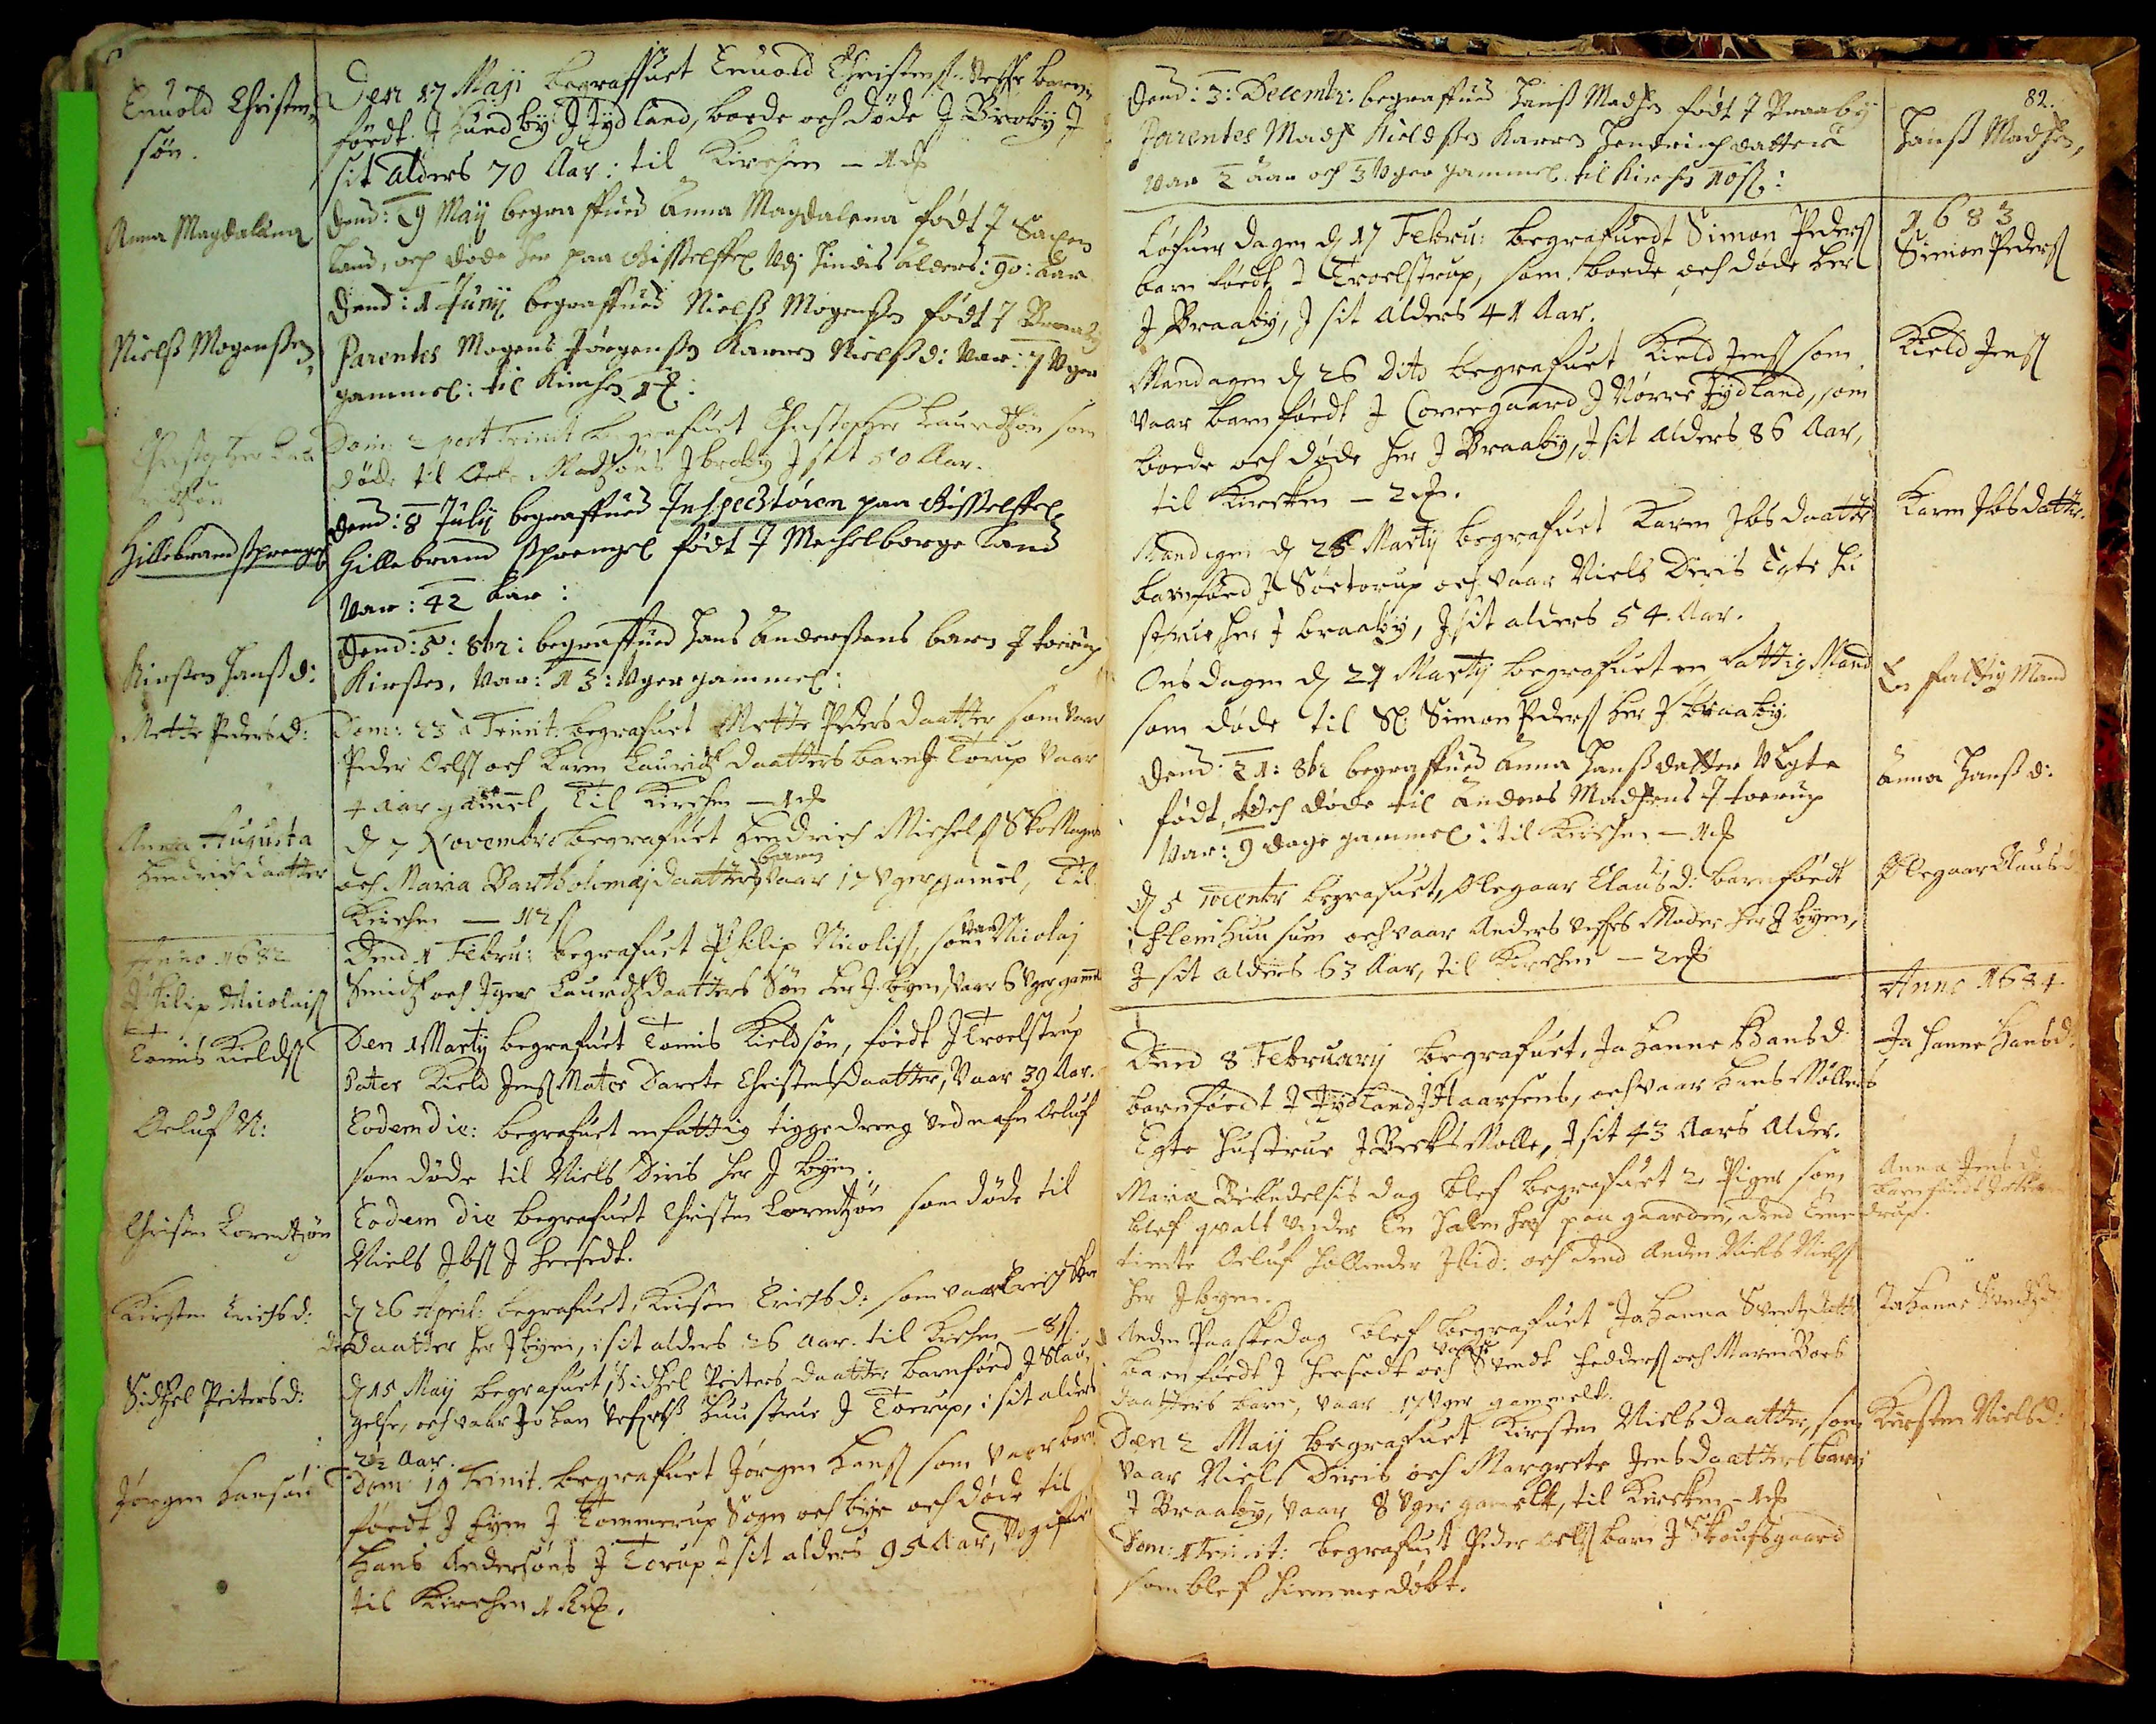Envold Christen¬
søn 
Den 17 May begraffuet Envold Christens: Veffer barn
føedt i Lundby J Jydland, boede och døde J Braaby. J
sit alders 70 Aar: til Kirchen 1 Mk.
Anna Magdalene
Dend: 29 May begraffued Anna Magdalene født J Saxens
Land, och døde her paa Gisselffel Udj hindis Alders: 90: Aar. 
Dend: 1 Juny begraffued Niels Mogensen født J Braaby
Niels Mogensen
Parentes Mogens Jørgensøn Karren NielsD: Var: 7 Uger 
gammel: til Kirchen 1 Mk
Christopher Laurid-
zøn
Dom 2 post Trinit begrafuet Christopher Lauridsøn som
døde til Oele Madzøns J Broby J sit 50 Aar.
Hillebrand Sprengel##
Dend: 8 July begraffued Inspecktøren paa Gisselffel
Hillebrand Sprengel født J Mechelborgs Land
var: 42 Aar:
Kirsten Hansd:
Dend: 5: 8br: begraffued Hans Andersøns Barn J Toerup;
Kirsten. Var: 13: Uger gammel:
Mette Pedersd:
Dom 23 á´Trinit: begrafuet Mette Pedersdaatter som vare
Peder Oels och Karen Lauridzdaatters Barn J Torup. Vaar
4 Aar gammel, til Kirchen 1Mk.
Anna## Augusta
Hendrichdaatter 
D. 7. November begrafuet Hendrich Michels SkoMager
Barn
och Maria Bartholemæj Daatters. Vaar 17 Uger gammel. Til
Kirchen – 12 Sk.
Anno 1682
Philip Nicolais
Den 1 Febru: begrafuet Philip Nicolis, som var Nicolaj
Smidz och Jnger Lauridzdaatters Søn her J Byen. Vaar 6 Uger Gammel.
Tomis Kields
Den 1 Marty begrafuet Tomis Kieldsøn, føedt J Troelstrup.
Pater Kield Jens, Mater Dorete Christensdaatter. Vaar 39 Aar.
Oeluf N:
Eodem die: begrafuet en fattig Tiggedreng ved Nafn Oeluf
som døde til Niels Diris her J Byen.
Christen Lorentzøn
Eodem die begrafuet Christen Lorentzøn som døde til
Niels Jbs J Hersedt.
Kirsten Erichsd:
D. 26 April: begraffuet Kirsten ErichsD: som vaar Erich ##
ders## Daatter her J byen, i sit alders 26 Aar, til Kirchen - 8 Sk:
Sidzel Pedersd. 
D. 15 May begrafuet, Sidzel Peitersdaatter barnføed J Slau¬
gelse, och vaar Johan Vesztes## Hustrue J Toerup, i J sit alders
20 ½ Aar.
Jørgen Hansøn
Dom: 19 Trinit. begrafuet Jørgen Hans som vaar barn
føedt J Fyen J Tommerup Sogn och bye och døde til
Hans Andersøns J Torup J sit alders 95 Aar, udgift
til Kirchen 1 Rmk.
Dend: 3: Decembr: begraffued Hans Madsen født J Braaby.
Parentes Mads Nielssen, Karen Hendrichdatter,
var 2 Aar och 3 Uger gammel, til Kirche 10 Sk:
82.
Hans Madsen
Løfensdagen d. 17 Febru: Begraffuedt Simon Peders
barn føedt J Troelstrup, som boede och døde her
J Braaby, J sit alders 41 Aar. 
1683
Simon Pedersøn
Mandagen d 26 dito begrafuet Kield Jens som
vaar barn føedt J Corregaard J Nørre Jydland, som
boede och døde her J Braaby, J sit alders 86 Aar,
til Kircken - 2 Mk.
Kield Jens
Mandagen d. 26 Marty begrafuet Karen Jbsdaatter
barnføed J Søetorup och Vaar Niels Diris Egte Hu
strue her J Braaby, J sit alders 54 Aar.
Karen Jbsdaatter.
Onsdagen d. 27 Marty begrafuet en fattig Mand
som døde til SL: Simon Peders her J Braaby.
En fattig Mand
Dend: 21: 8br. . Begrafued Anna Hansdatter Uegte
født. och døde til Anders Madsens J Toerup,
Var: 9 Dage gammel: til Kirchen - 1 Mk.
Anna Hansd:
D. 5 10cembr. begrafued Ølegaar Clausd: Barnføedt
J  FlemmHuusum##  och vaar Anders Veffrs Moeder her J byen,
J sit alders 63 Aar, til Kirchen - 2 Mk.
Ølegaar Clausd:
Den 8 February begrafuet, Johanne HansD
barnføedt J Jydland J Haarsens, och vaar Hans Møllers
Egte Hustrue J BecksMølle, J sit 43 Aars Alder.
Anno 1684
Johanne Hansd:
Maria Bebudelsis Dag blef begrafuet 2 Piger som
blef qvalt under En Halm Hees## her paa gaarden, dend Eene
tiente Oeluf hollænder Jbid: och dend anden Niels Niels
her J byen.
Anna Jensd:
barnføedt i Otted##
drup##
Anden Paaskedag blef begrafuet Johanna Sventzdatter
vaar
barnføedt J Hersedt och Svends Fedders och Maren Boes
daatters barn, Vaar 17 Uger gammelt.
Johanna Sventzd:##
Den 2 May begrafuet Kirsten Nielsdaater, som
Vaar Niels Diris och Margrete Jensdaatters barn
J Braaby, Vaar 8 Uger gammelt, til Kircken 1 Mk.
Dom: 1.Trinit: begrafuet Peder Oels Barn J Skoufsgaard
som blef hiemmedøbt.
Kirsten Nielsd:
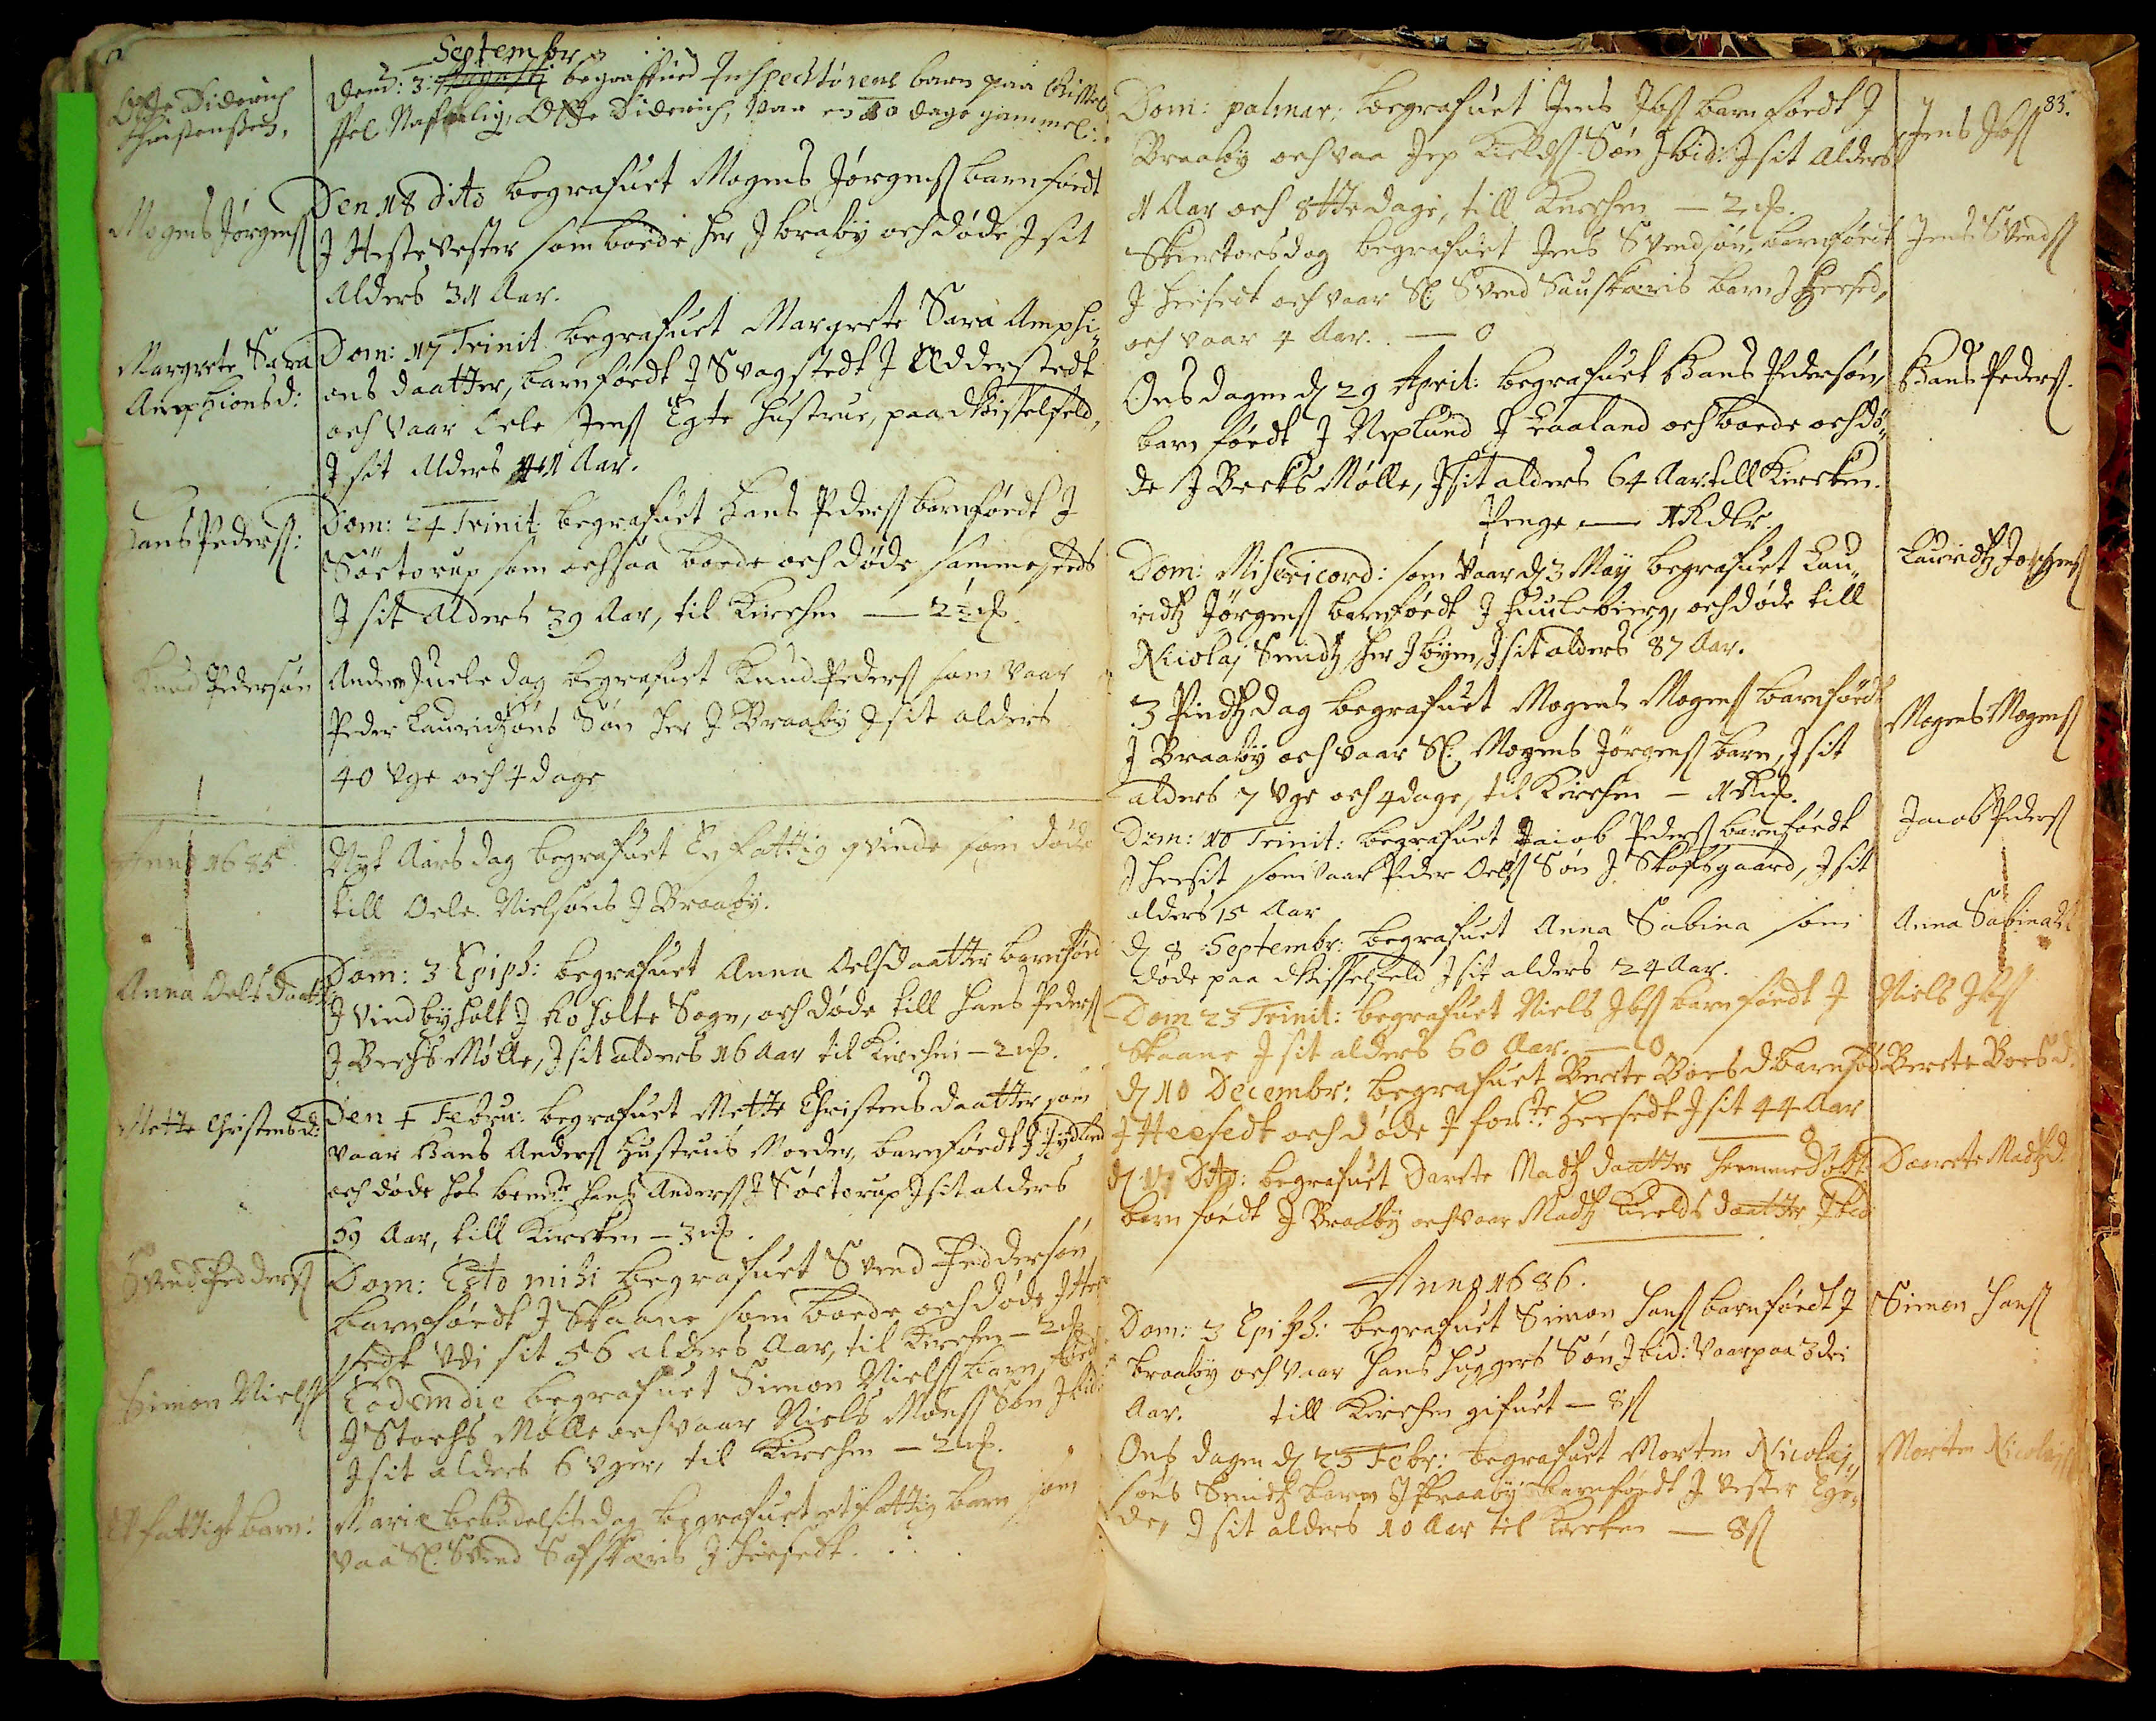Otte Diderich
Christenssen,
September
Dend: 3: Augustj begraffued Inspecktørens barn paa Gissel
feld, Naffnlig Otte Diderich, var en 10 Dage gammel:
Mogens Jørgens
Den 18 dito begrafuet Mogens Jørgens barnføedt
J Heste## Vester som boede her J Broby och døde J sit
alders 31 Aar. 
Margrete Sara
Amphionsd: 
Dom: 17 Trinit begrafuet Margrete Sara Amphi¬
ons daatter barnføedt J Svagstedt J Ædderstedt##
och Vaar Oele Jens Egte hustrue paa Gisselfeld,
J sit alders 41## Aar.
Hans Peders:
Dom: 24 Trinit: begrafuet Hans Peders barnføedt J
Søetorup som ocksaa boede och døde sammesteds
J sit alders 39 Aar, til Kirchen - 2 ½ Mk
Knud Pedersøn
Anden Jueledag begrafuet Knud Peders som Vaar
Peder Lauridzøns Søn her J Braaby, J sit aldris
40 Uge och 4 dage. 
Anno 1685
Nyt Aars Dag begrafuet En fattig qvinde, som døde
till Oele Nielsøns J Braaby.
Anna Oelsdaatter
Dom: 3 Epiph: begrafuet Anna Oelsdaatter barnføed
J Vindbyholt J Roholte Sogn, och døde till Hans Peders
J Bechsmølle, J sit alders 16 Aar, til Kirchen -2 Mk.
Mette Christensd:
Den 4 Febru: begrafuet Mette Christensdaatter, som
Vaar Hans Anders Hustrues Moeder, barnføedt J Jydland
och døde hos bemde## Hans Anders J Søetorup J sit alders
69 Aar, till Kircken - 3 Mk. 
Svend Fedders
Dom: Esto mihi begrafuet Svend Feddersøn
barnføedt J Skaane som boede och døde J Her¬
sedt. Udi sit 56 alders Aar, til Kirchen - 2 Mk.
Simon Niels
Eodem die begrafuet Simon Niels barnføedt
J Stochs Mølle och Vaar Niels Mons Søn Jbid,
J sit alders 6 Uger til Kirchen - 2 Mk.
Et fattigt Barn.
Marie Bebudelsis Dag begrafuet eet fattig barn som
Vaar Sl. Svend Safskæris J Hirsedt:
Dom: Palmar: begrafuet Jens Jbs barnføedt J
Braaby och var Jep Kields Søn Jbid: J sit alders
1 Aar och 8tte dagi, till Kirchen - 2 Mk.
83.
Jens Jbs
Skeirtorsdag begrafuet Jens Svendsøn, barnføedt
J Hirsedt och Vaar SL: Svend Sauskeiris## barn J Hersed,
och vaar 4 Aar -0
Jens Svends
Onsdagen d 29 April: begrafuet Hans Pedersøn,
barnføedt J Neplund J Laaland och boede och dø¬
de J Becks Mølle, J sit alders 64 Aar. till Kircken.
Penge - 1 Rdlr.
Hans Peders
Dom: Misericord: som Vaar d 3 May begrafuet Lau¬
ridtz Jørgens barnfødt J Fuulebierg och døde till
Nicolaj Smidtz her J byen, J sit alders 87 Aar.
Lauridtz Jørgens
3 Pindsedag begrafuet Mogens Mogens barnføedt
J Braaby och vaar SL Mogens Jørgens barn, J sit
alders 7 Uge och 4 Dage, til Kirchen -1 Rmk.
Mogens Mogens
Dom: 10 Trinit: begrafuet Jacob Peders barnføedt
J Hersit som vaar Peder Oels Søn J Skofsgaard, J sit
alders 15 Aar 
Jacob Peders
D. 8 Septembr: begrafuet Anna Sabina som
døde paa Gisselfeld J sit aldiers 24 Aar
Anna Sabina##
Dom 23 Trinit: begrafuet Niels Jbs barnføedt J
Skaane J sit alders 60 Aar - 0
Niels Jbs
D. 10 Decembr: begrafuet Berete BoesD: barnføed
J Heesedt och døde J forte## Heesedt J sit 44 Aar
Berete Boesd.
D. 17 Dito: begrafuet Dorete Madsdaatter hiemme Døbt
barn føedt J Braaby och vaar Madz Kields daatter Jbid.
Daarete Madzd:
Anno 1686.
Dom: 3 Epiph: begrafuet Simon Hans barnføedt J
Braaby och Vaar Hans Huggers Søn Jbid: Vaar paa 3die
Aar - till Kirchen gifuet - 8 Sk
Simon Hans
Onsdagen dj 23 Febr: begrafuet Morten Nicolaj¬
sens## Smidtz barn J Braaby, barnføedt J Vester Ege¬
de, J sit alders 10 Aar, til Kircken - 8 Sk
Morten Nicolajsøn##